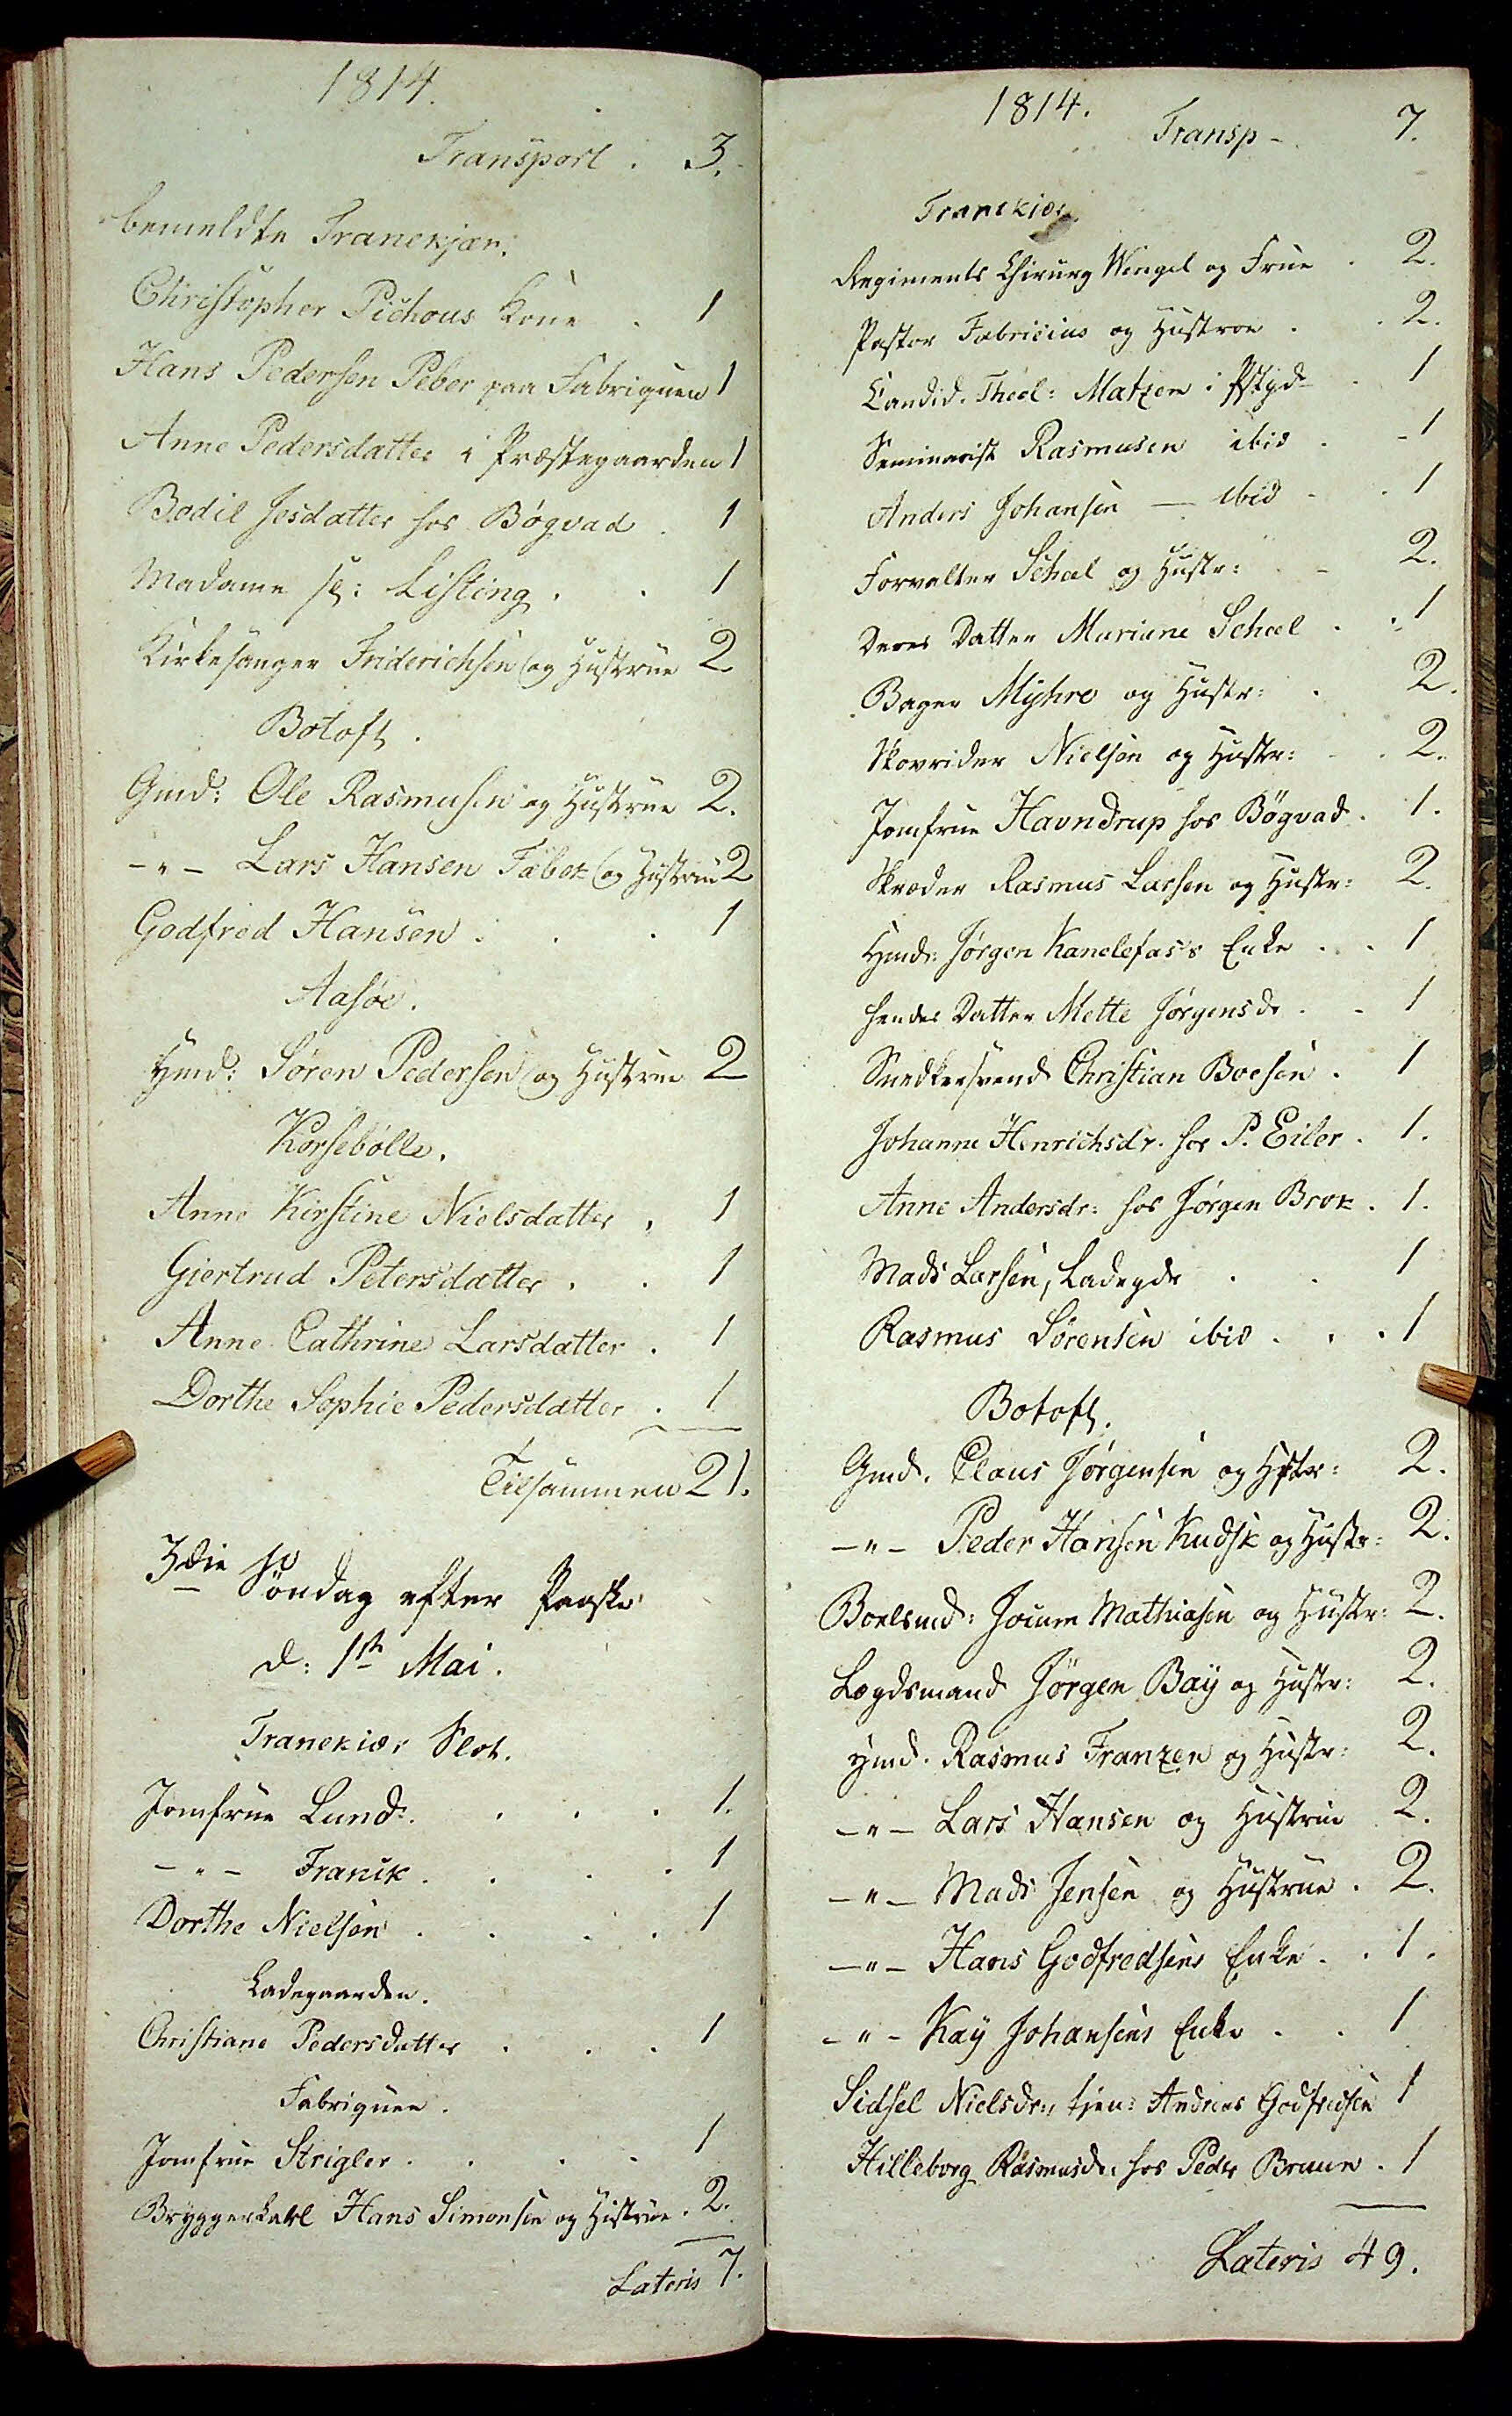1814.
Transport  . 3.
bemeldte Tranekjær.
Christopher Pichous Kone .1
Hans Pedersen Peber paa Fabriquen 1
Anne Pedersdatter i Præstegaarden 1
Bodil Jesdatter hos Bøgvad . 1
Madame sl: Listing . . 1
Kirkesanger Friderichsen og Hustrue 2
Botoft
Gmd: Ole Rasmusen og Hustrue 2.
-"- Lars Hansen Fæbek og Hustrue 2
Godfred Hansen . . . 1
Aasøe.
Hmd: Søren Pedersens og Hustrue 2
Korsebølle.
Anne Kirstine Nielsdatter . 1
Gjertrud Petersdatter . . 1
Anne Cathrine Larsdatter . 1
Dorthe Sophie Pedersdatter . 1
Tilsammen 21.
3die Søndag efter Paaske.
d. 1ste Mai.
Tranekiær Slot.
Jomfrue Lund . . . . 1.
-"- Franck . . . . 1
Dorthe Nielsen . . . . 1
Ladegaarden.
Christiane Pedersdatter . . . 1
Fabriquen.
Jomfrue Strigler . . . . 1
Bryggerkarl Hans Simonsen og Hustrue . 2.
Lateris 7.
1814.
Transp - 7.
Tranekiær
Regiments Chirurg Wengel og Frue . 2.
Pastor Fabricius og Hustroe . . 2.
Candid. Theol: Matzen i Pstgdr . 1
Seminarist Rasmusen ibid . . 1
Anders Johansen - ibid . . 1
Forvalter Scheel og Hustr: . - 2.
Deres Datter Mariane Scheel . . 1
Bager Myhre og Hustr: . 2.
Skovrider Nielsen og Hustr: . . 2.
Jomfrue Havndrup hos Bøgvad. 1.
Skræder Rasmus Larsen og Hustr: 2.
Hmd. Jørgen Kanelefas's Enke . . 1
hendes Datter Mette Jørgensdr . . 1
Snedkersvende Christian Boesen . 1.
Johanne Henrichsdr. hos P. Eiler . 1.
Anne Andersdr: hos Jørgen Brok . 1.
Mads Larsen, Ladegdr . . 1
Rasmus Sørensen ibid . . 1
Botoft
Gmd. Claus Jørgensen og Hstr: 2.
-"- Peder Hansen Kudsk og Hustr: 2.
Boelsmd: Jocum Mathiasen og Hustr: 2.
Lægdsmand Jørgen Bay Hustr: 2.
Hmd. Rasmus Franzen og Hustr: 2.
-"- Lars Hansen og Hustrue 2.
-"- Mads Jensen og Hustrue . 2.
-"- Hans Godfredsens Enke . . 1.
-"- Kay Johansens Enke . . 1
Sidsel Nielsdr:, tjen: Andreas Godfredsen 1
Hilleborg Rasmusdr: hos Peder Bruun . 1
Lateris 49.
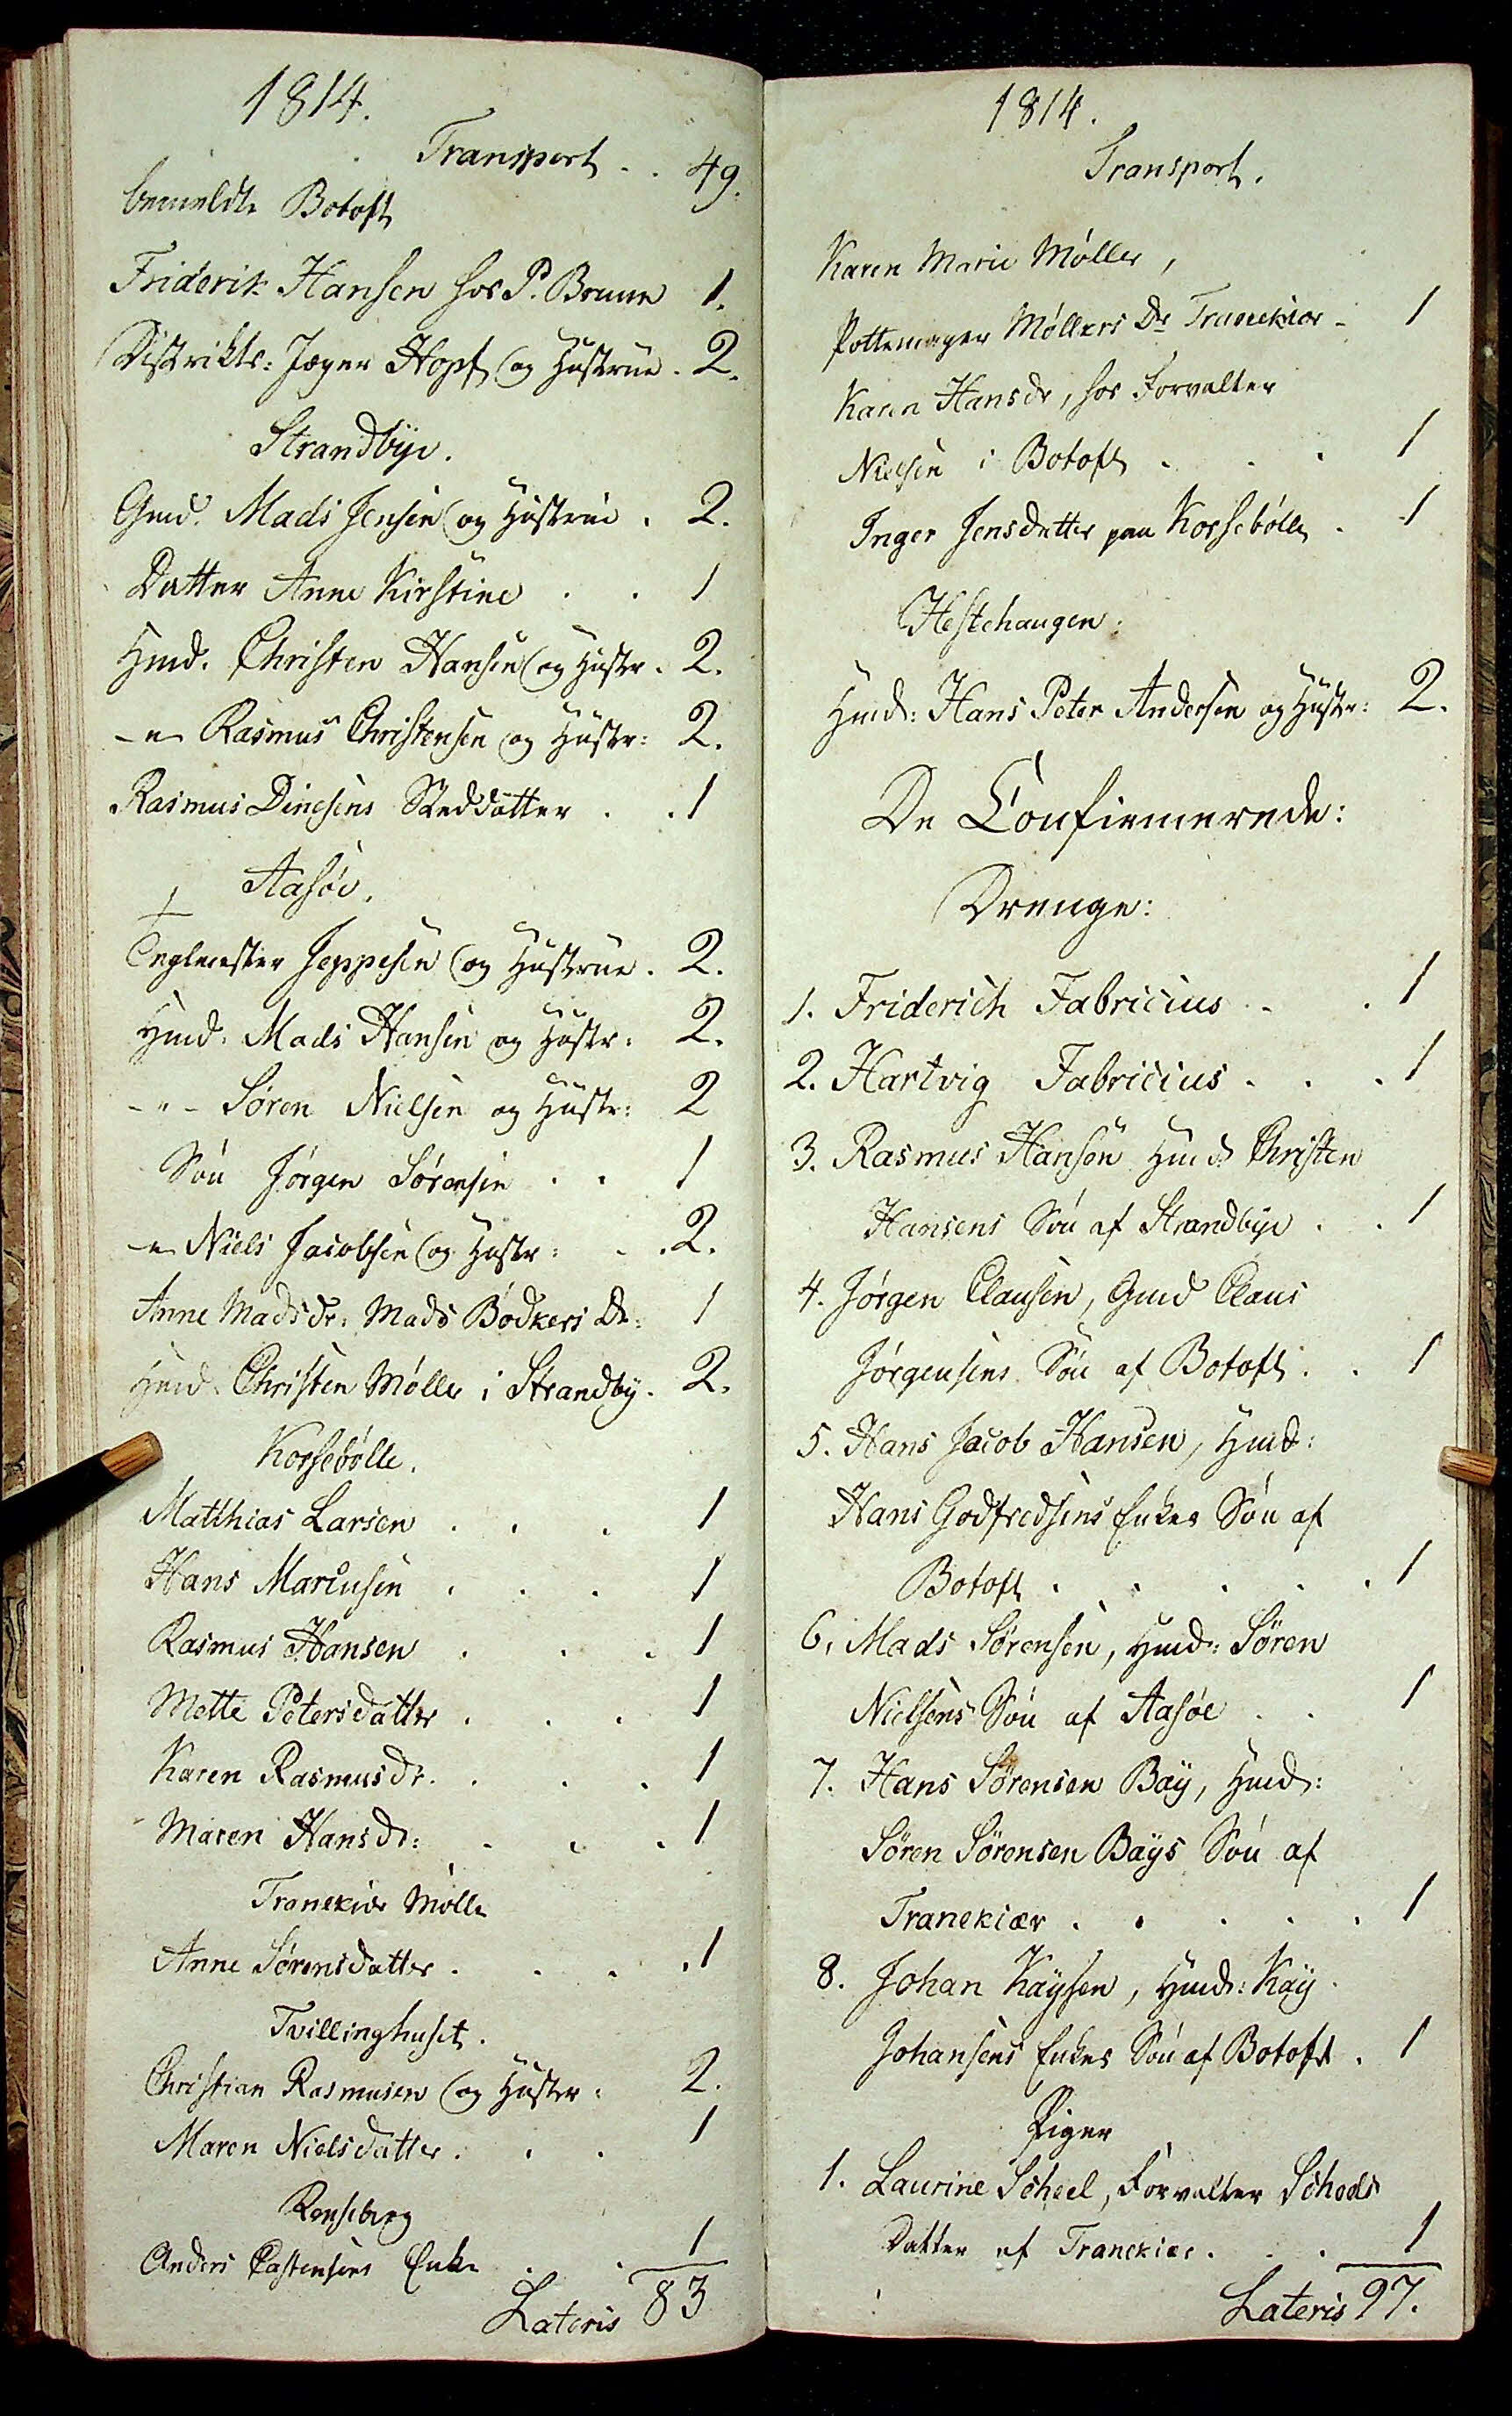1814.
Transport . . 49.
bemeldte Botoft
Friderik Hansen hos P. Bruun 1.
Distrikts: Jæger Hopf og Hustrue . 2.
Strandbye.
Gmd. Mads Jensen og Hustrue . 2.
Datter Anne Kirstine . . 1
Hmd: Christen Hansens og Hustr. 2.
-"- Rasmus Christensen og Hustr: 2.
Rasmus Dinesens Steddatter . . 1
Aasøe.
Teglmesater Jeppesen og Hustrue . 2.
Hmd. Mads Hansen og Hustr: 2.
-"- Søren Nielsen og Hustr: 2
Søn Jørgen Sørensen . . 1
-"- Niels Jacobsen og Hustr: . . 2.
Anne Madsdr: Mads Bødkers Dr: 1
Hmd. Christen Møller i Srandby . 2.
Korsebølle.
Matthias Larsen . . . 1
Hans Marcusen . . . 1
Rasmus Hansen . . . 1
Mette Petersdatter . . . 1
Karen Rasmusdr . . . 1
Maren Hansdr: . . .1
Tranekiær Mølle
Anne Sørensdatter . . . . 1
Tvillinghuset
Christian Rasmusen og Hustr: 2.
Maren Nielsdatter . . . 1
Renseberg
Anders Castensens Enke . . 1
Lateris 83
1814.
Transport.
Karen Marie Møller,
Pottemager Møllers Dr Tranekiær - 1
Karen Hansdr, hos Forvalter
Nielsen i Botoft . . . 1
Inger Jensdatter paa Korsebølle . .1
Hestehaugen
Hmd: Hans Peter Andersen og Hustr: 2.
De Confirmerede:
Drenge:
1. Friderich Fabricius . . . 1
2. Hartvig Fabricius . . . 1
3. Rasmus Hansen Hmd: Christen
Hansens Søn af Strandbye . . 1
4. Jørgen Clausen, Gmd Claus
Jørgensens Søn af Botoft . . 1
5. Hans Jacob Hansen, Hmd:
Hans Godfredsens Enkes Søn af
Botoft . . . . . 1
6. Mads Sørensen, Hmd: Søren
Nielsens Søn af Aasøe . . 1
7. Hans Sørensen Bay, Hmd:
Søren Sørensen Bays Søn af
Tranekiær . . . . . 1
8. Johan Kaysen, Hmd: Kay
Johansens Enkes Søn af Botoft . 1
Piger
1. Laurine Scheel, Forvalter Scheels
Datter af Tranekiær . . . 1
Lateris 97.